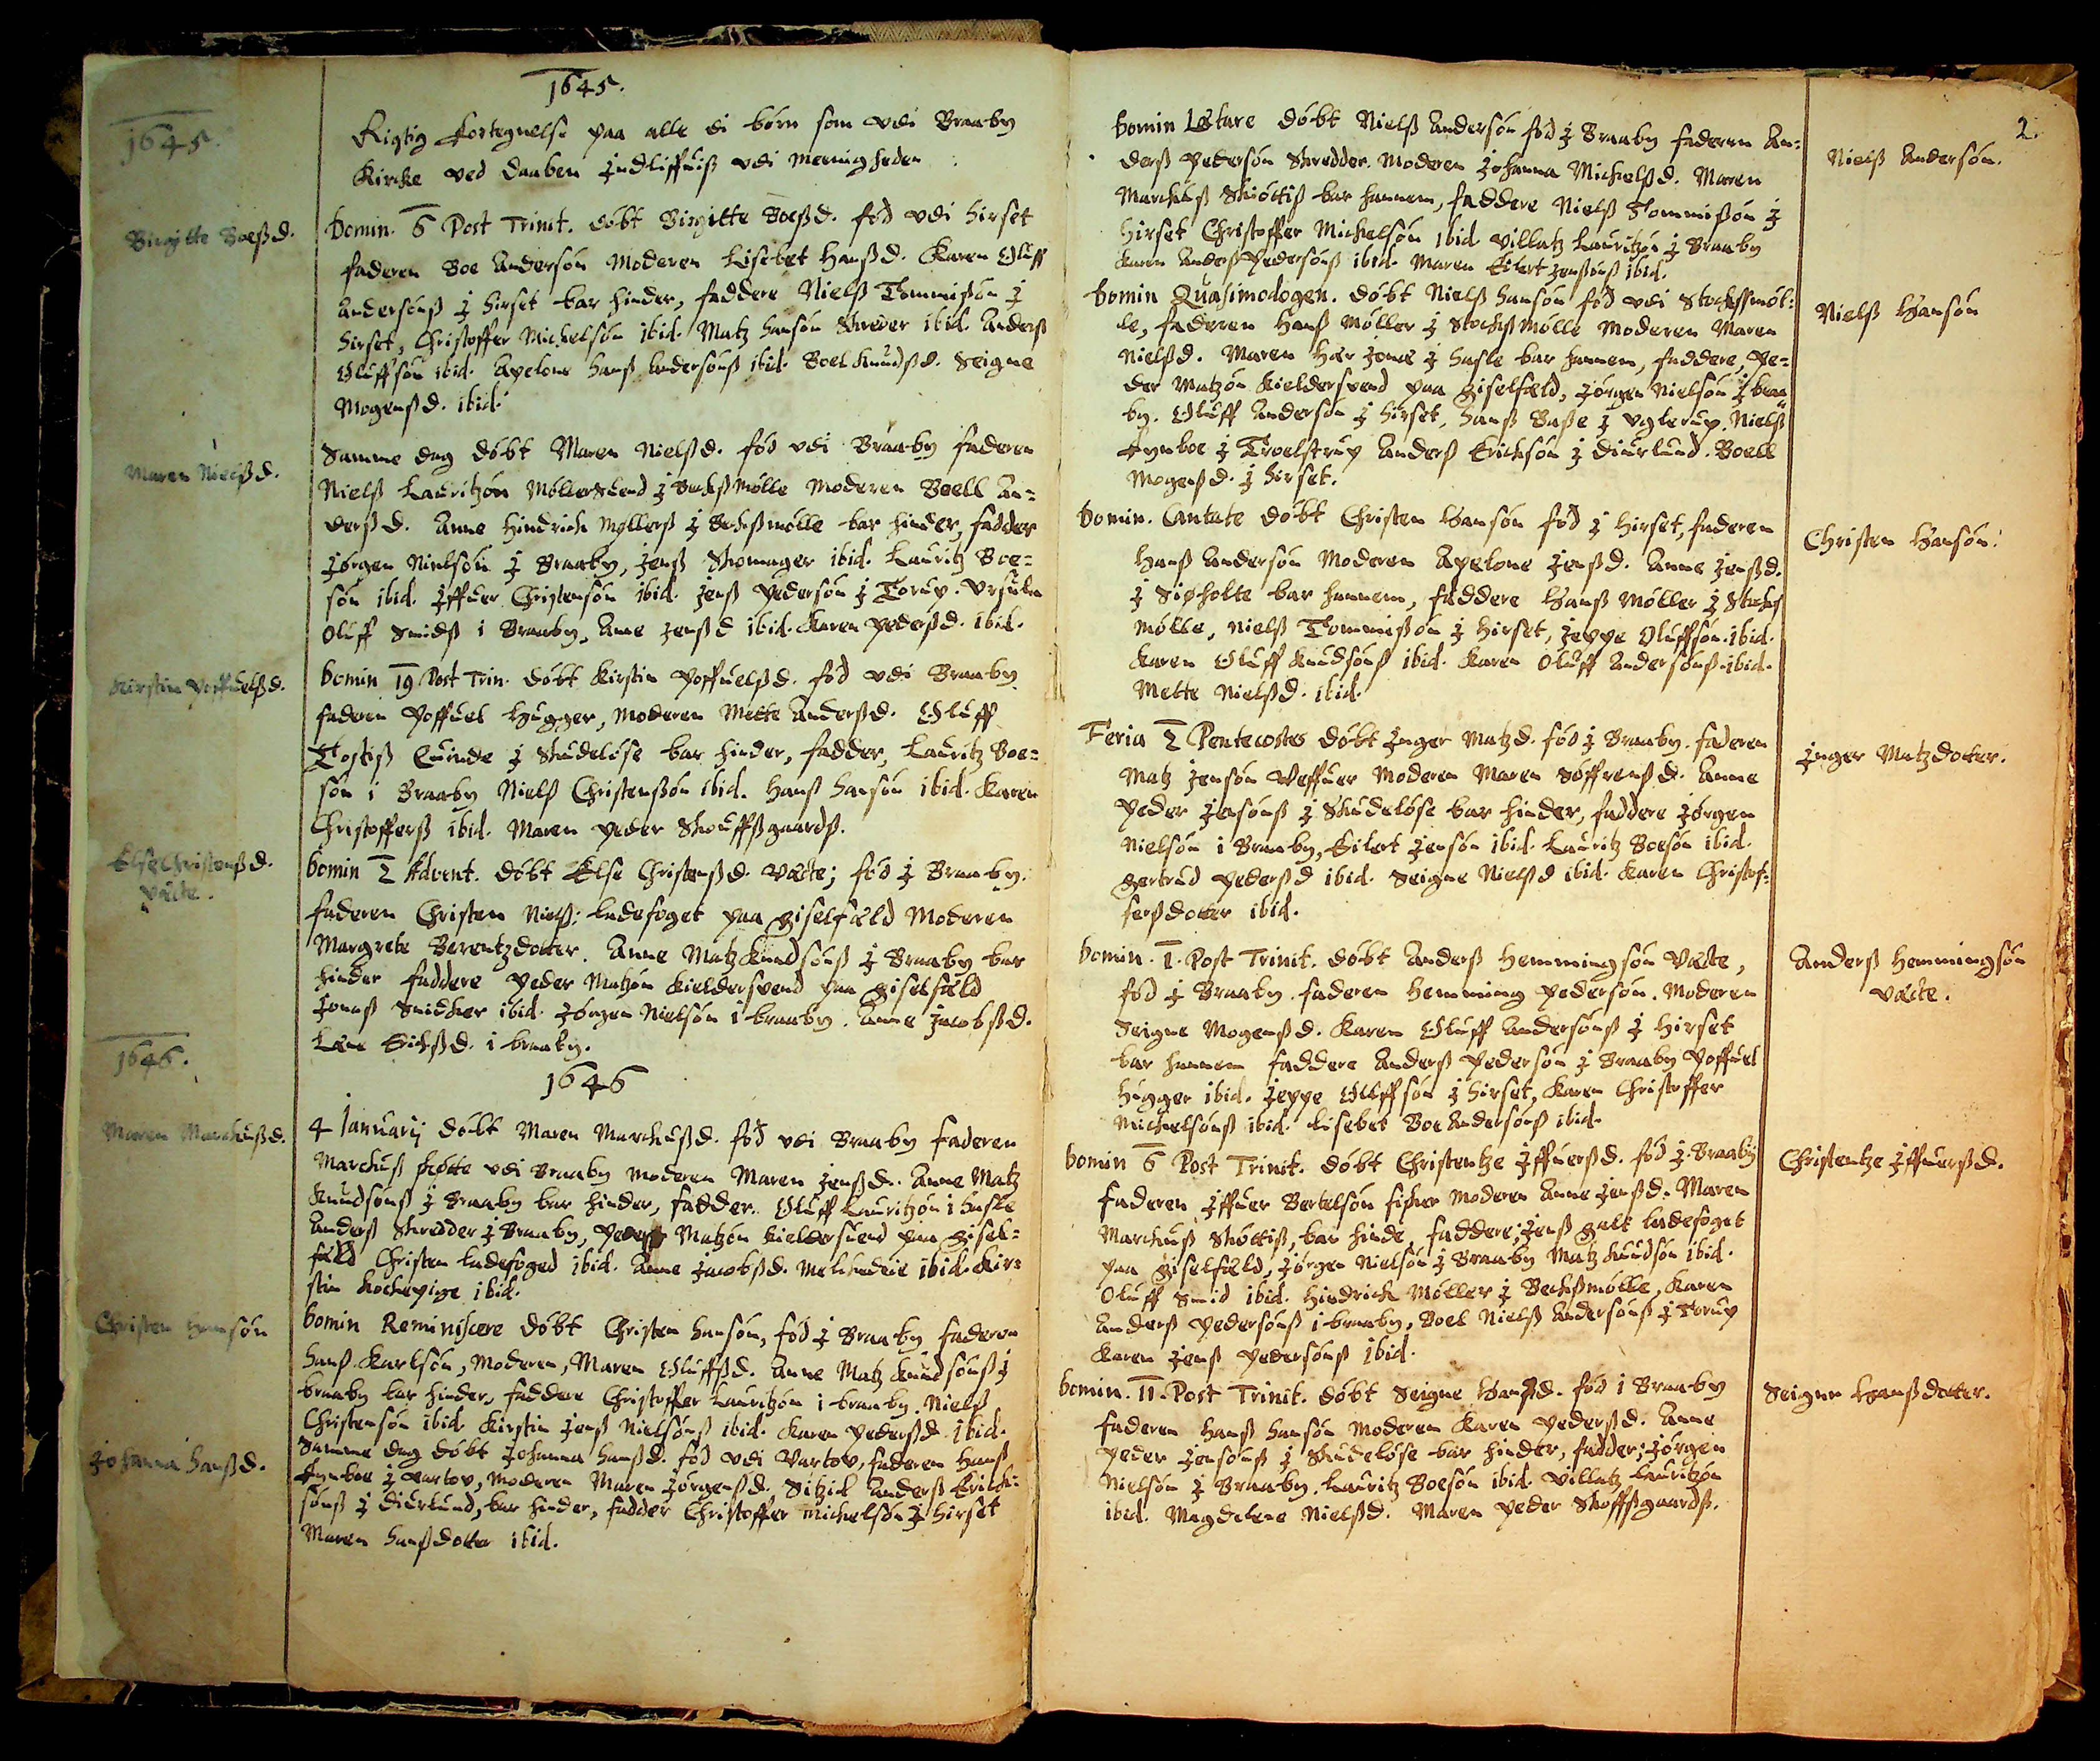1645.
1645.
Rigtig fortegnelse paa alle di børn  som udi Braaby 
Kirke ved Daaben indliffuis udi Menigheden 
Birgitte Boesd. 
Domin 6 Post Trinit. døbt Birgitte Boesd. fød udi Hirset 
Faderen Boe Andersøn Moderen Lisebet Hansd. Karen Oluff 
Andersøns I Hirset bar hinder, Faddere, Niels Thommisøn i 
Hirset, Christoffer Mickelsøn ibid. Matz Hansøn Skreder ibid.  Anders 
Oluffsøn ibid. Apelone Hans Andersøns ibid. Boel  Knudsd, Seigne 
Mogensd. ibid:
Maren Nielsd. 
Samme dag døbt Maren Nielsd. fød udi Braaby, Faderen
Niels Lauritzøn Møllersvend I Becksmølle Moderen Boell An¬
dersd. Anne Hendrick Møllers I Becksmølle bar hinder,  Fadder 
Jørgen Nielsøn I Braaby, Jens Skomager ibid. Lauritz  Boe¬
søn ibid Iffuer Christensøn ibid. Jens Pedersøn I Torup. Ursula 
Oluff Smids i Braaby, Ane Jensd. ibid. Karen Pedersd. ibid. 
Kirstin Poffuelsd.
Domin 19 Post Trin. døbt Kirstin Poffuelsd. fød udi Braaby. 
Faderen Poffuel Hugger, Moderen Mette Andersd. Oluff
Tostis## Quinde I Skudeløse bar hinder, Fadder, Lauritz Boe¬
søn i Braaby Niels Christensøn ibid. Hans Hansøn ibid. Karen 
Christoffers ibid. Maren Peder Skouffsgaards. 
Else Christensd.
Uæcte 
Domin 2 Advent. døbt Else Christensd. Uæcte; fød I Braaby:
Faderen Christen Niels: Ladefoget paa Giselfæld Moderen 
Margrete Berentzdatter. Anne Matz Knudsøns I Braaby bar 
hinder Faddere Peder Matzøn Kieldersvend paa Giselfæld
Jonas Snidker ibid. Jørgen Nielsøn i Braaby. Anne Jacobsd, 
Læne Erichsd. i Braaby. 
1646.
Maren Marckusd. 
1646
4 january døbt Maren Marckusd. fød udi Braaby, Faderen 
Marchus Skøete udi Braaby Moderen Maren Jensd. Anne Matz 
Knudssøn I Braaby bar hinder, Fadder Oluff Lauritzøn i Hasle 
Anders skrædder I Braaby, Peder Matzøn Kieldersvend paa Giesel¬
fæld, Christen Ladefoged ibid. Anne Jacobsd.  Mats ## ibid. Kirs¬
sten Kockepige ibid. 
Christen Hansøn 
Domin. Reminiscere døbt Christen Hansøn, fød I Braaby Faderen 
Hans Karlsøn, Moderen, Maren Oluffsd. Anne Matz Knudsøns I
Braaby bar hinder, Faddere. Christoffer Lauritzøn i Braaby. Niels
Christensøn ibid. Kirstin Jens Nielsøns ibid. Karen Pedersd. ibid. 
Johanne Hansd. 
Samme dag døbt Johanne Hansd. fød udi Vartov, Faderen Hans
Fynboe I Vartov, Moderen Maren Jørgensd, Sitzil Anders Erick¬
søns I Diurlund, bar hinder, Fadder Christoffer Mickelsøn I Hirset 
Maren Hansdotter ibid. 
Domin Lætare døbt Niels Andersøn fød I Braaby. Faderen An¬
ders Pedersøn Skrædder. Moderen Johanne Michelsd. Maren 
Marchus## Skiøctis bar hannem, Faddere Niels Thommisøn I 
Hirset, Christoffer Michelsøn ibid. Villatz Lauritzøn I Braaby
Karen Anders Pedersøns ibid, Maren Erhart Jenssøns ibid.
2.
Niels Andersøn
Domin Quasinmodogen. døbt Niels Hansøn fød udi Stocksmøl¬
le, Faderen Hans Møller I Stocksmølle, Moderen Maren
Nielsd. Maren Hær Jonæ I Hasle bar hannem, Faddere  Pe¬
der Matzøn Kieldersvend paa Giselfæld, Jørgen Nielsøn I Braa¬
by, Oluff Andersøn I Hirset, Hans Gase I Uglerup. Niels  
Fynboe I Troelstrup Anders Ericksøn I Diurlund. Boell 
Mogensd. I Hirset. 
Niels Hansøn 
Domin. Cantate døbt Christen Hansøn fød I Hirset, Faderen 
Hans Andersøn Moderen Apelone Jensd. Anne Jensd.
I Siøholte bar hannem, Faddere Hans Møller I Stataf##
Mølle, Niels Thommisøn I Hirset, Jeppe Oluffsøn. ibid.
Karen Oluff Knudsøns ibid. Karen Oluff Andersøns ibid. 
Mette Nielsd. ibid. 
Christen Hansøn 
Feria 2 Pentecostes døbt Inger Matzd. fød I Braaby, Faderen 
Matz Jensøn Veffuer Moderen Maren Søffrensd. Anne
Peder Jensøns I Skudeløse bar hinder, Faddere Jørgen
Nielsøn i Braaby, Eilert Jensøn ibid. Lauritz Boesøn ibid.
Gertrud Pedersd. ibid. Seigne Nielsd. ibid. Karen Christof¬
fersdotter ibid.
Inger Matzdatter 
Domin 1. Post Trinit. døbt Anders Hemmingsøn Uæcte,
fød I Braaby. Faderen Hemming Pedersøn. Moderen 
Seigne Mogensd. Karen Oluff Andersøns I Hirset
bar hannem Faddere Anders Pedersøn I Braaby Poffuel 
Hugger ibid. Jeppe Oluffsøn I Hirset Karen Christoffer 
Mickelsøns ibid. Lisebet Boe Andersøns ibid. 
Anders Hemmingsøn  
Uæcte 
Domin 6 Post Trinit. døbt Christentze Jffuersd. fød I Braaby. 
Faderen Iffuer Bertelsøn Fisker Moderen Anne Jensd. Maren
Marckus Shøttis, bar hinde, Faddere; Jens Galt Ladefoged 
paa Giselfæld, Jørgen Nielsøn I Braaby Matz Kuudsøn ibid. 
Oluff Smid ibid. Hindrick Møller I Beckssmølle, Karen 
Anders Pedersøns i Braaby. Boel Niels Andersøns I Torup
Karen Jens, Pedersøns ibid.
Christentze Iffuersd
Domin 11. Post Trinit. døbt Seigne Hansd. fød i Braaby 
Faderen Hans Hansøn Moderen Karen Pedersd. Anne 
Peder Jensøn I Skudeløse bar hinder, Fadder; Jørgen 
Nielsøn I Braaby. Lauritz Boesøn ibid. Villatz Lauritzøn 
ibid. Magdelene Nielsd. Maren Peder Skoffsgaards.
Seigne Hansdatter